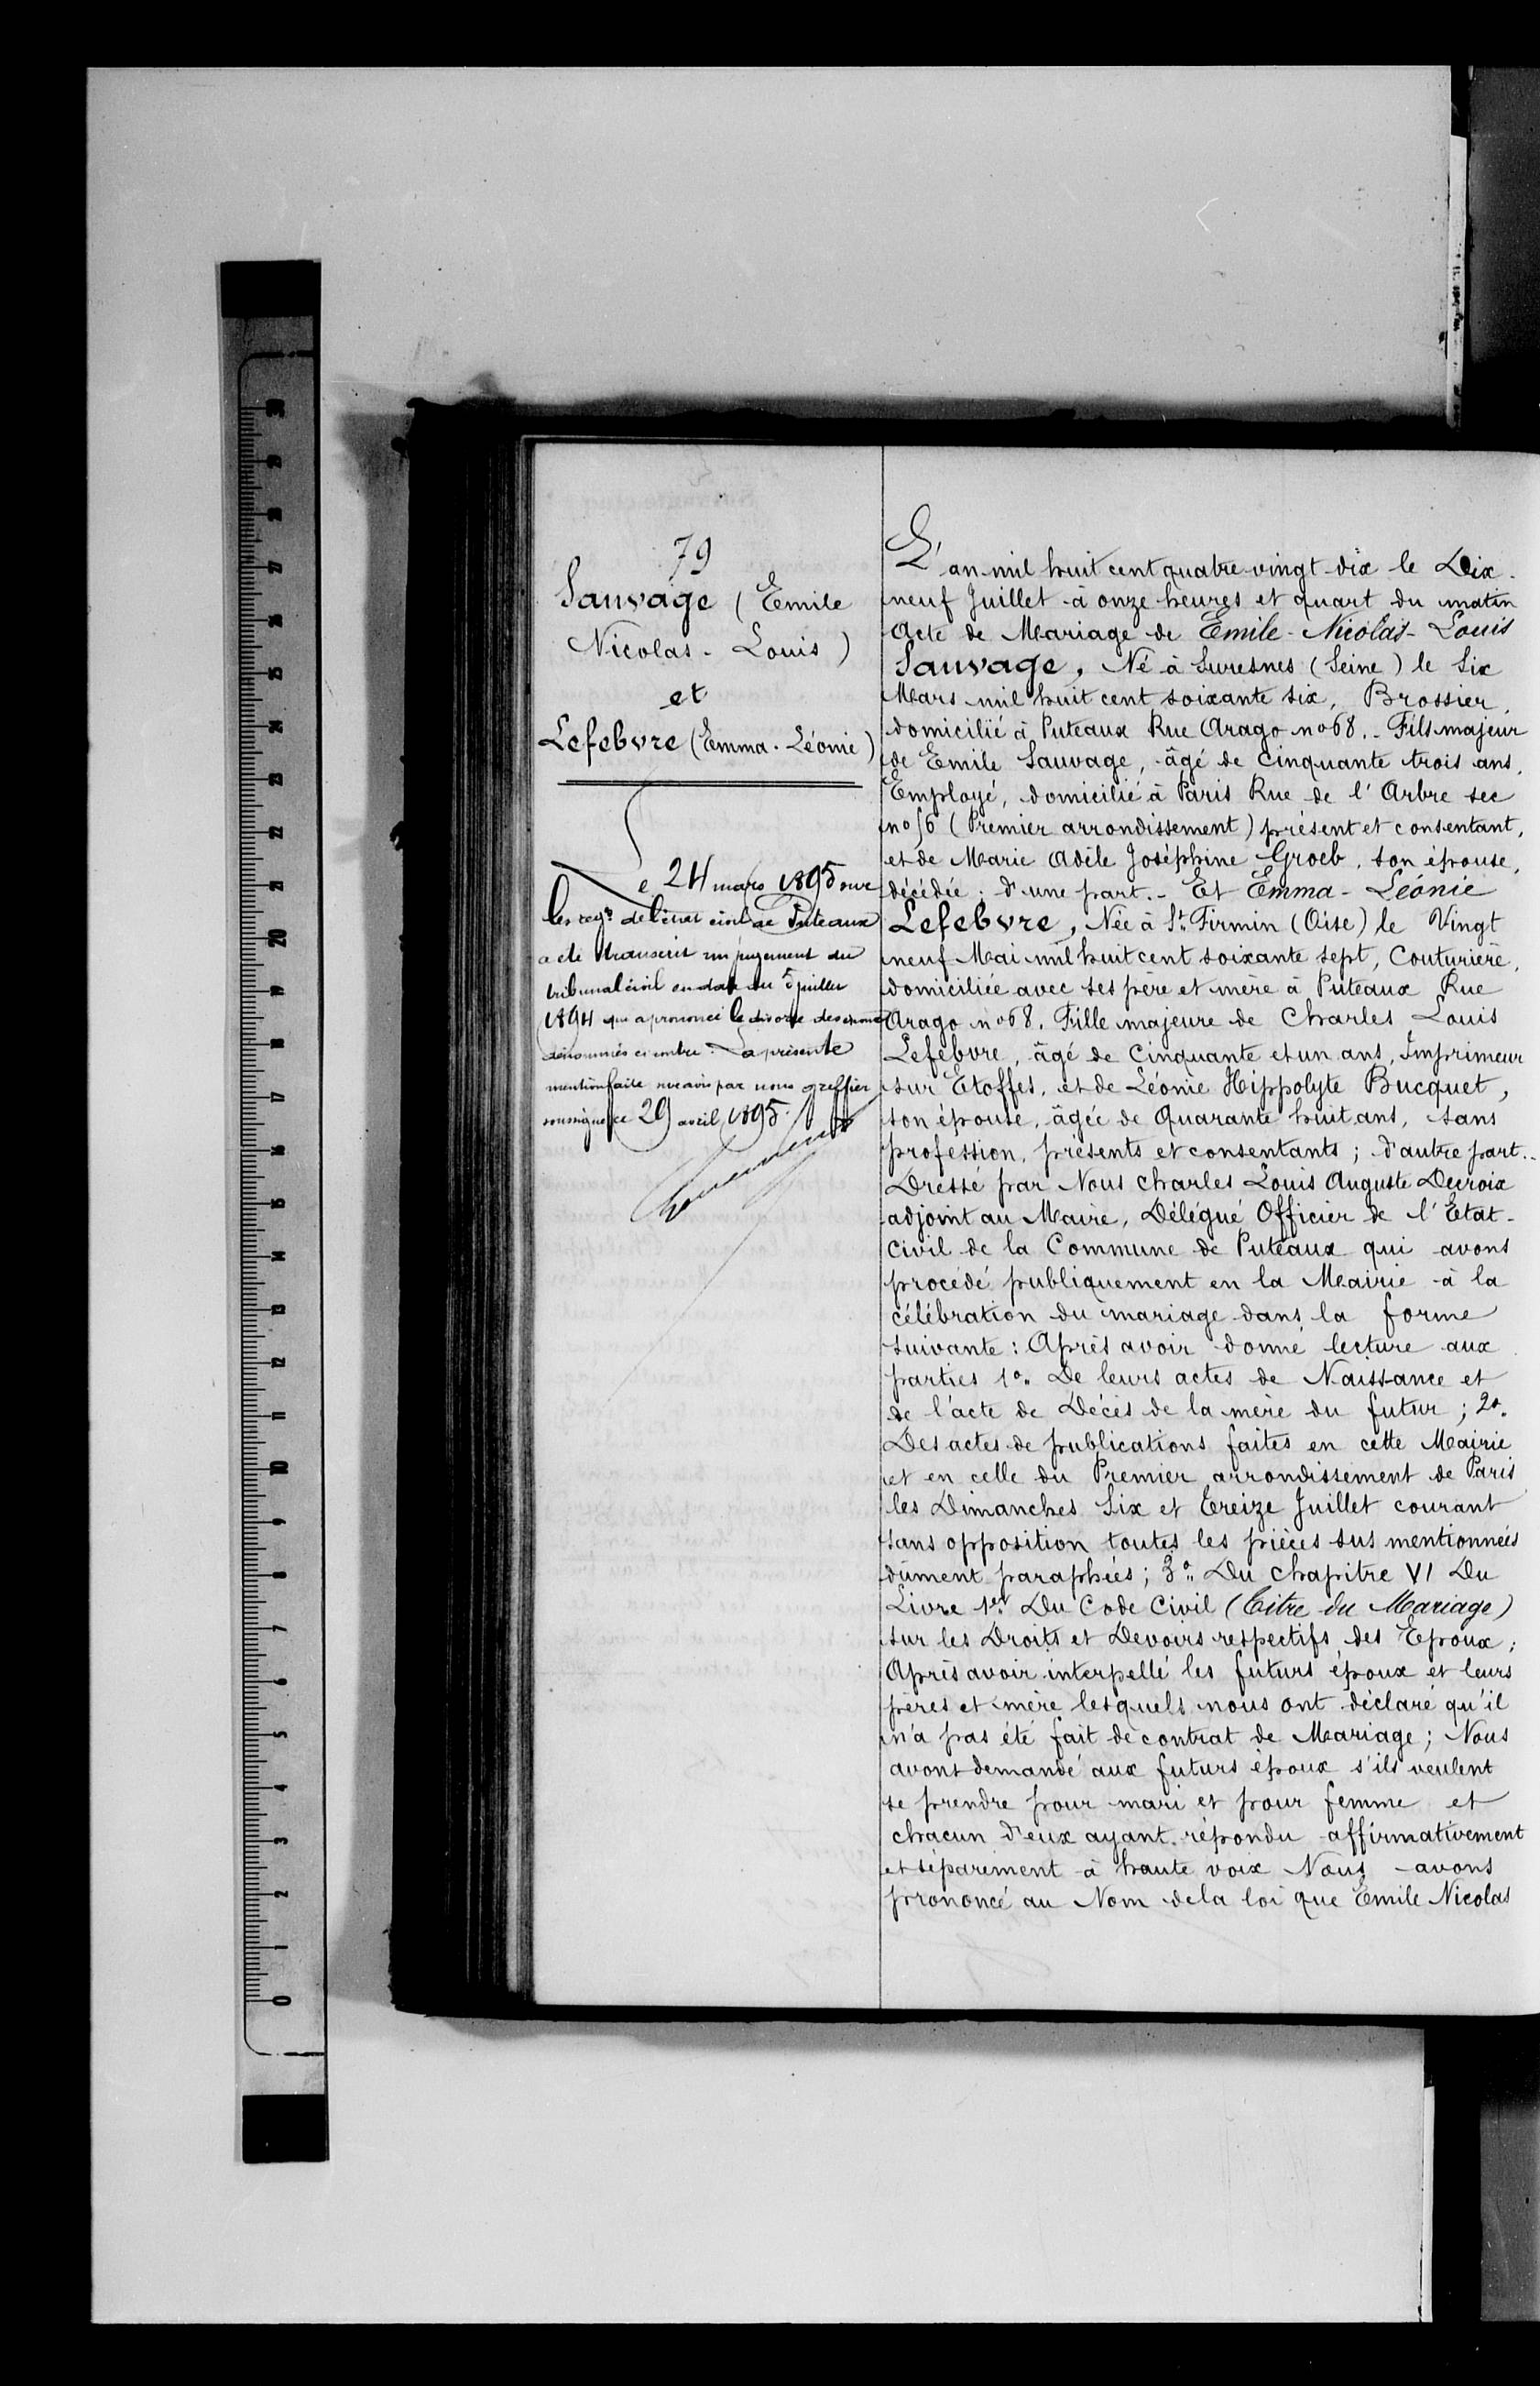79
Sauvage (Emile
Nicolas-Louis)
et
Lefebvre (Emma-Léonie)
L'an mil huit cent quatre vingt-dix le dix-
neuf juillet à onze heures et quart du matin
acte de Mariage de Emile Nicolas Louis
Sauvage, Né à Suresnes (Seine) le six
Mars mil huit cent soixante six, Brossier
domicilié à Puteaux Rue Arago n°68.-Fils majeur
de Emile Sauvage, âgé de cinquante trois ans.
Employé, domicilié à Paris Rue de l'Arbre sec
n°56 (Premier arrondissement) présent et consentant,
et de Marie Adèle Joséphine Groeb, son épouse,
décédée d'une part. -Et Emma Léonie
Lefebvre, Née à St Firmin (Oise) le Vingt
neuf Mai mil huit cent soixante sept, Couturière,
domiciliée avec ses père et mère à Puteaux Rue
Arago n°68. Fille Majeure de Charles Louis
Lefebvre, âgé de Cinquante et un ans, Imprimeur
sur Etoffes, et de Léonie Hippolyte Bucquet,
son épouse, âgée de Quarante huit ans, sans
profession, présents et consentants; d'autre part.-
Dressé par Nous Charles Louis Auguste Decroix
adjoint au Maire, Délégué Officier de l'Etat.
Civil de la Commune de Puteaux qui avons
procédé publiquement en la Mairie à la
célébration du mariage dans la forme
suivante: Après avoir donné lecture aux
parties 1°: De leurs actes de Naissance et
de l'acte de décès de la mère du futur; 2°
Des actes de publications faites en cette Mairie
et en celle du Premier arrondissement de Paris
les Dimanches Six et Treize juillet courant
sans opposition toutes les pièces sus mentionnées
dûment paraphées; 3°: Du chapitre VI Du
Livre 1er Du Code Civil (Titre du Mariage)
sur les Droits et Devoirs respectifs des Epoux;
Après avoir interpellé les futurs époux et leurs
pères et mère lesquels nous ont déclaré qu'il
n'a pas été fait de contrat de Mariage; Nous
avons demandé aux futurs époux s'ils veulent
se prendre pour mari et pour femme et
chacun d'eux ayant répondu affirmativement
et séparément à haute voix Nous avons
prononcé au Nom de la loi que Emile Nicolas
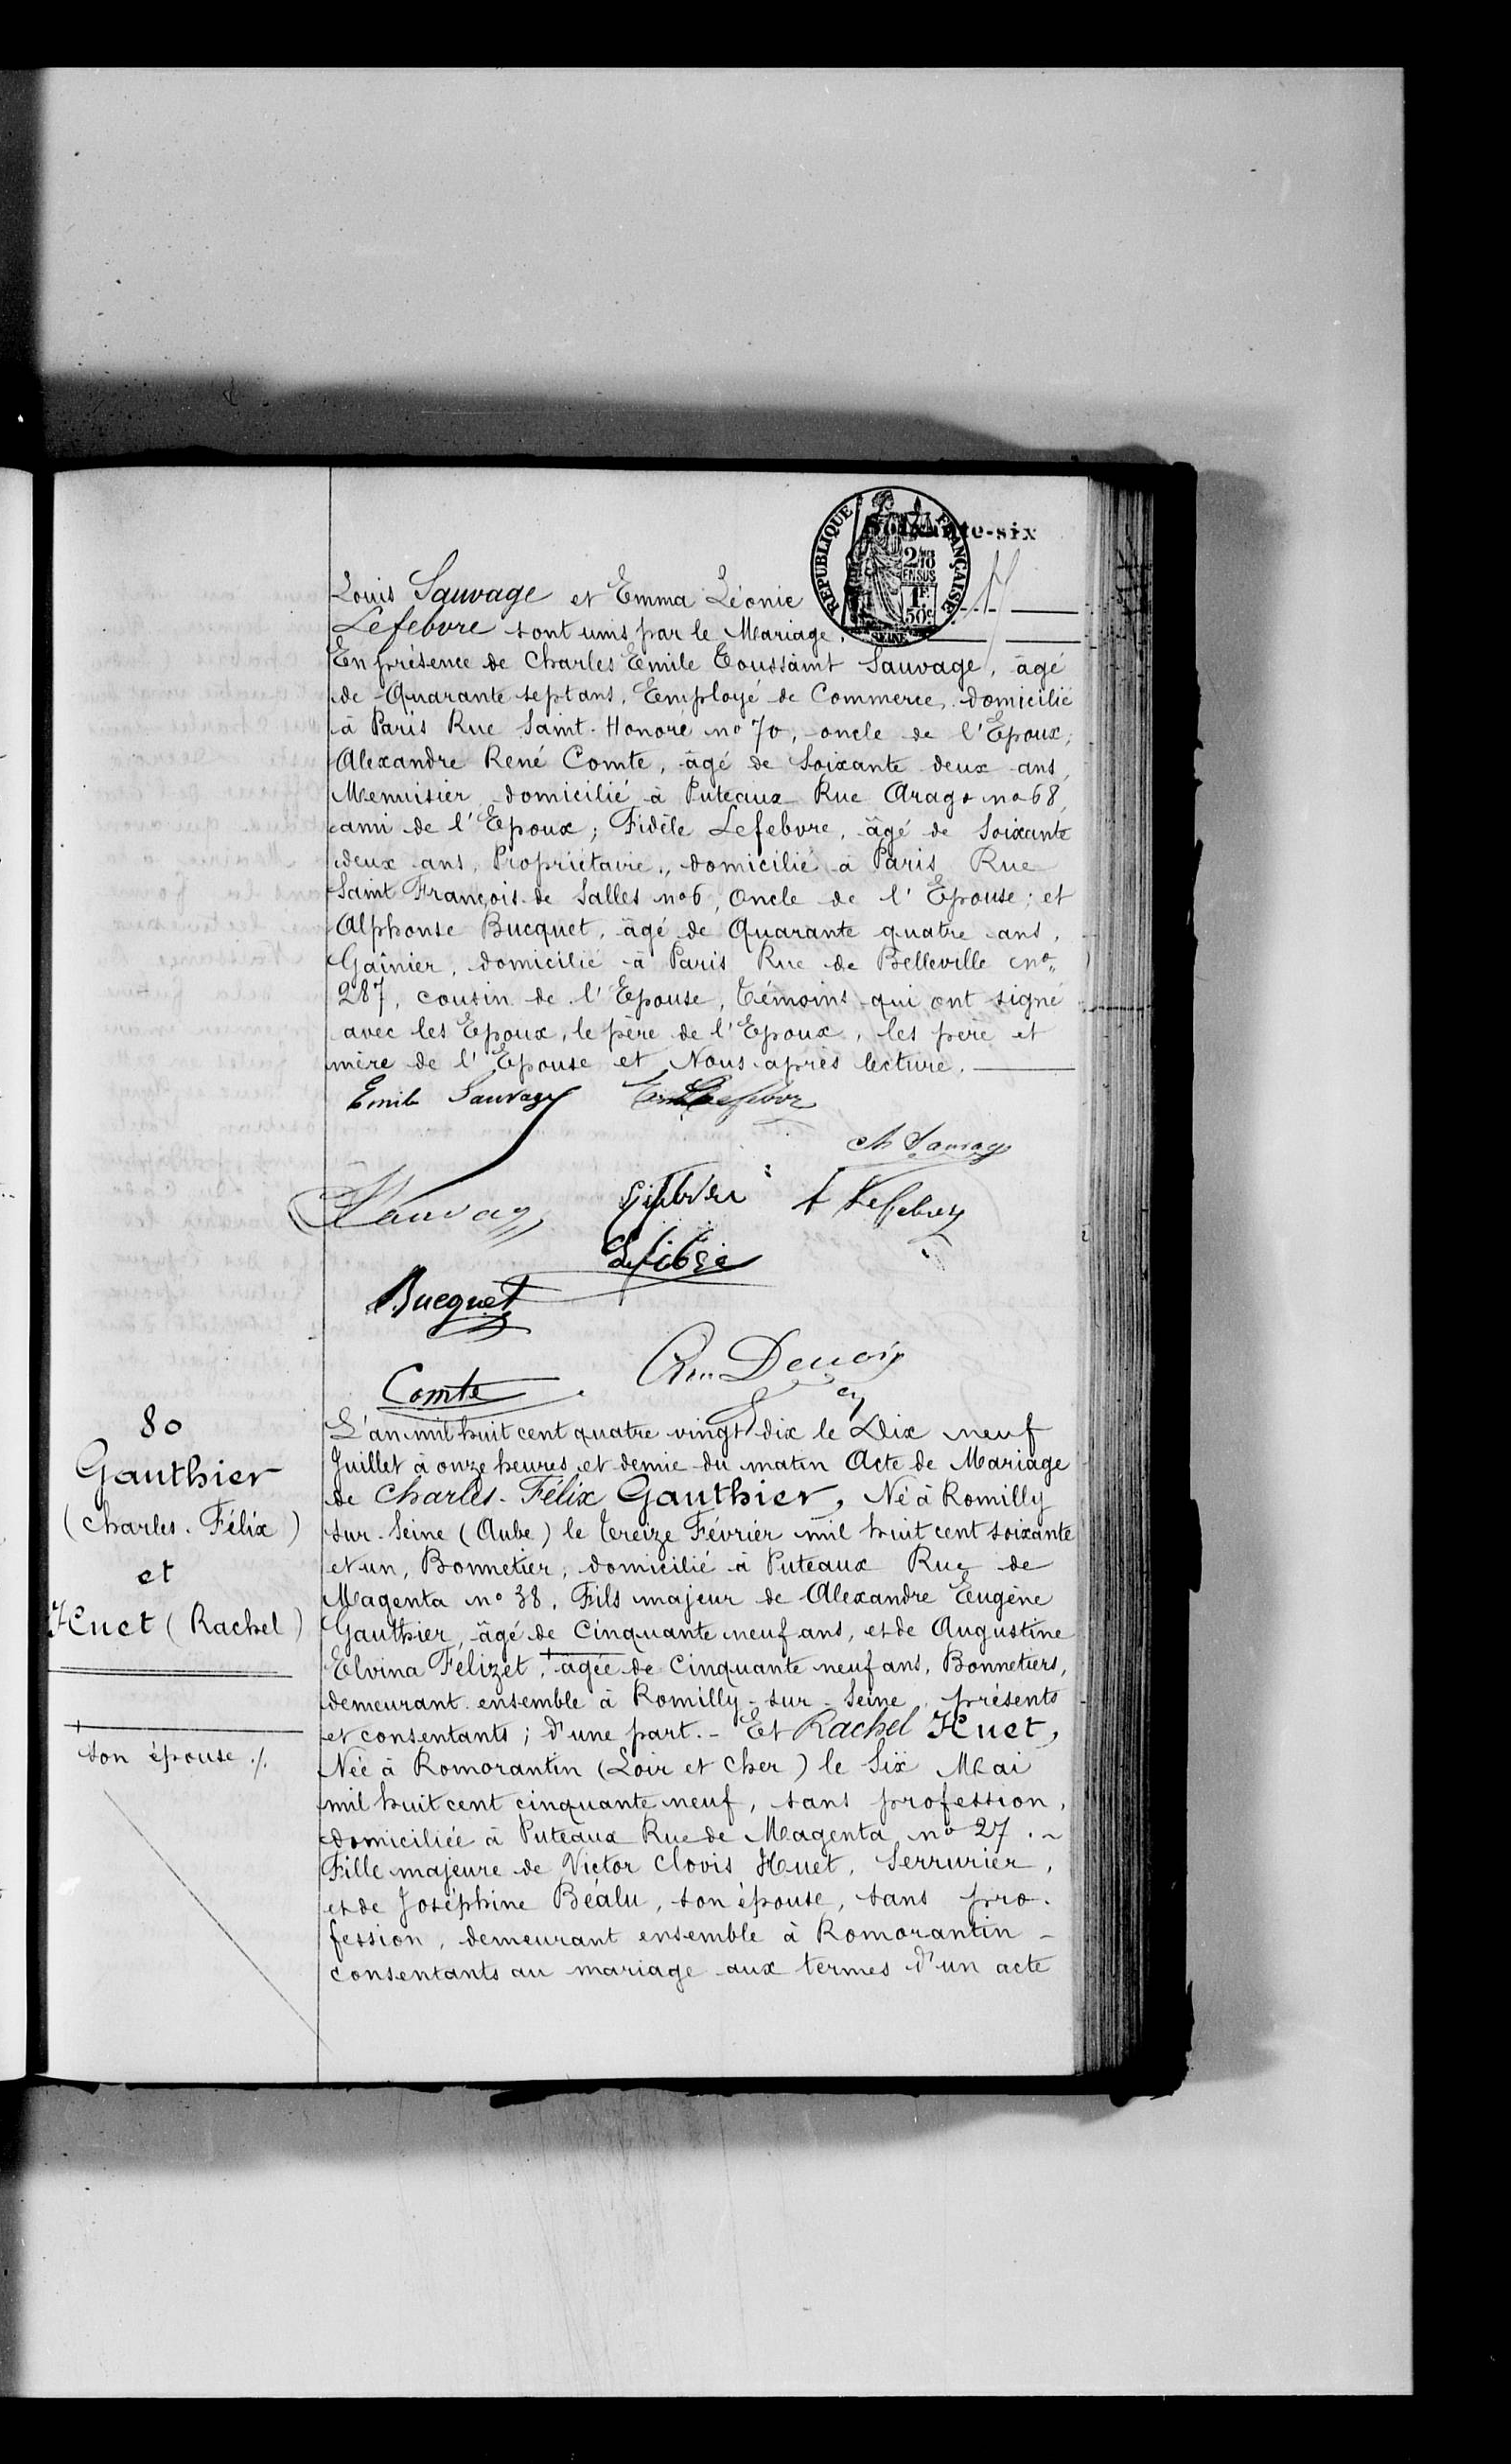Louis Sauvage et Emma Léonie
Lefebvre sont unis par le Mariage,
En présence de Charles Emile Toussaint Sauvage, âgé
de quarante sept ans. Employé de commerce, domicilié
à Paris Rue Saint Honoré n°70, oncle de l'Epouse
Alexandre René Comte, âgé de soixante deux ans
menuisier domicilié à Puteaux Rue Arago n°68,
ami de l'Epoux; Fidèle Lefebvre, âgé de soixante
deux-ans. Propriétaire, domicilié à Paris, Rue
Saint François de Salles n°6, oncle de l'Epouse; et
Alphonse Bucquet, âgé de quarante quatre ans,
Gainier, domicilié à Paris Rue de Belleville n°
287, cousin de l'Epouse, témoins qui ont signé
avec les Epoux, le père de l'Epoux, les père et
mère de l'Epouse et Nous après lecture.-
80
Gauthier
(Charles Félix)
et
Huet (Rachel)
L'an mil huit cent quatre-vingt-dix le dix neuf
juillet à onze heures et demie du matin Acte de Mariage
de Charles Félix Gauthier, Né à Romilly
sur Seine (Aube) le treize Février mil huit cent soixante
et un, Bonnetier, domicilié à Puteaux Rue de
Magenta n°38. Fils majeur de Alexandre Eugène
Gauthier âgé de cinquante neuf ans, et de Augustine
Elvina Félizet, âgée de cinquante neuf ans, Bonnetiers
demeurant ensemble à Romilly-sur-Seine présents
et consentants; d'une part.-Et Rachel Huet,
Née à Romorantin (Loir et Cher) le six Mai
mil huit cent cinquante neuf, sans profession,
domiciliée à Puteaux Rue de Magenta n°27 .-
Fille majeure de Victor Clovis Huet, serrurier,
et de Joséphine Béalu, son épouse, sans pro-
fession, demeurant ensemble à Romorantin -
consentants au mariage aux termes d'un acte